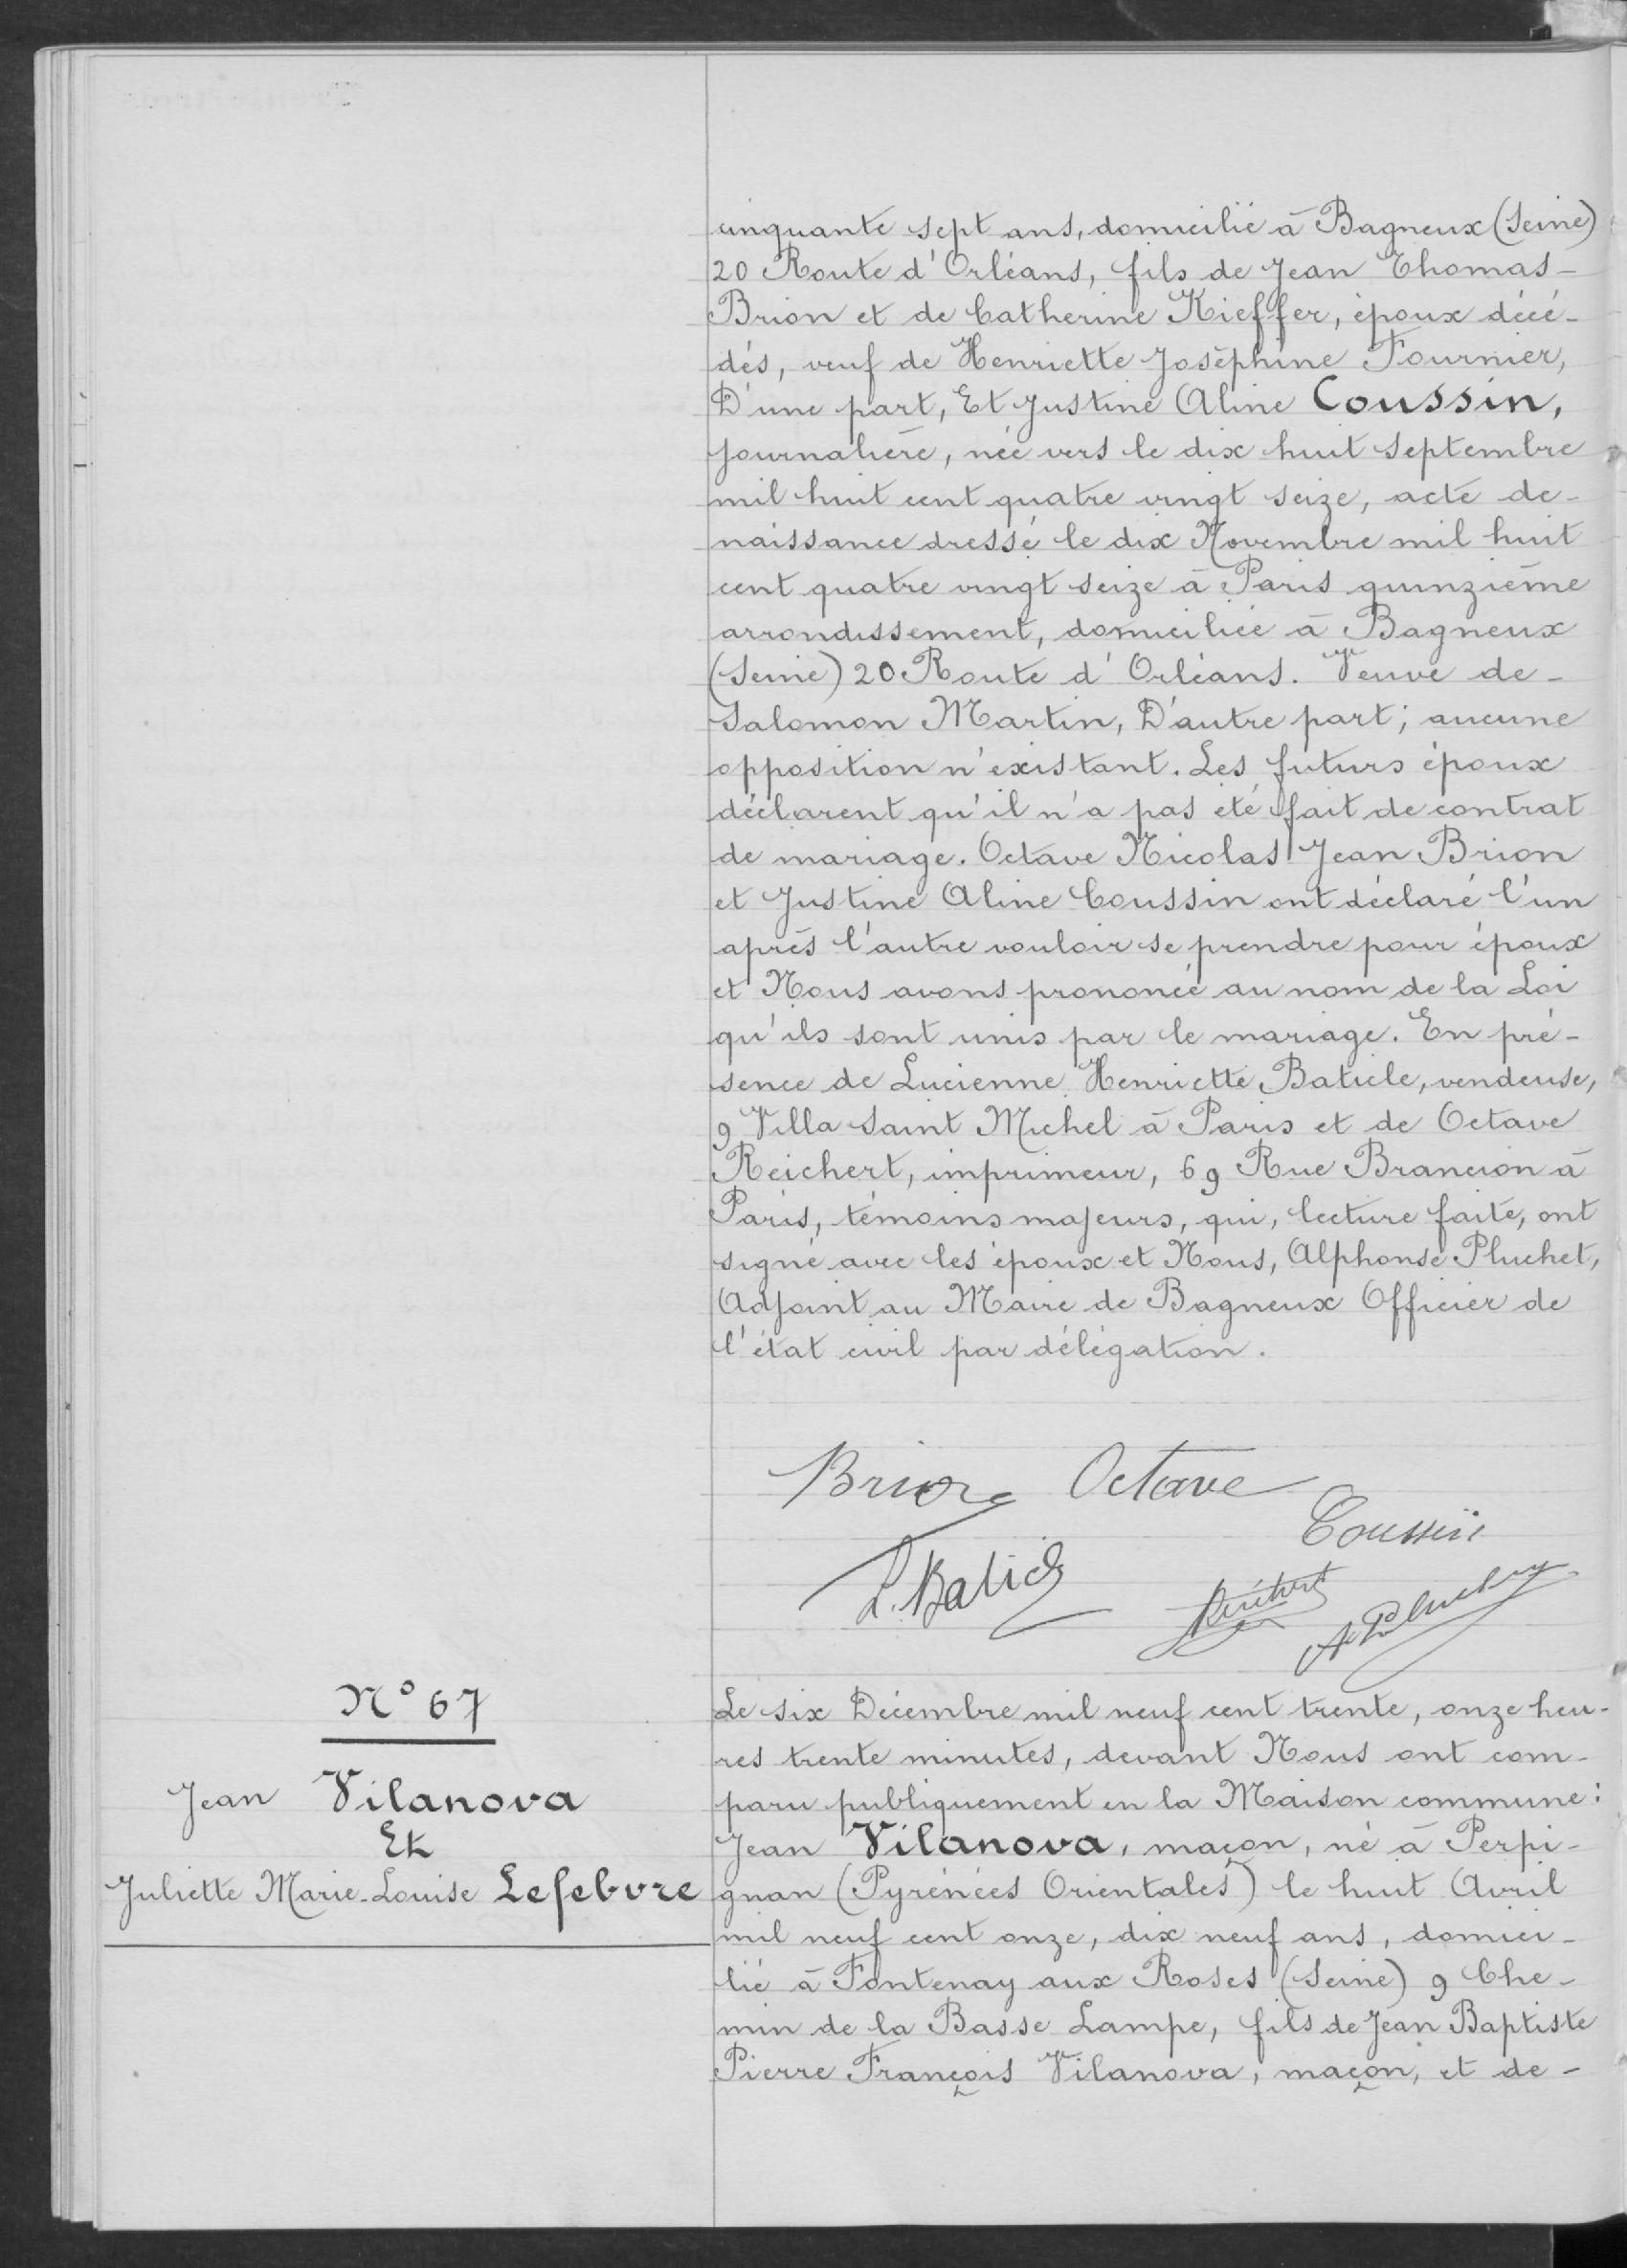cinquante sept ans, domicilié à Bagneux (Seine)
20 Route d'Orléans, fils de Jean Thomas -
Brion et de Catherine Kieffer, époux déc-
dés, veuf de Henriette Joséphine Fournier,
D'une part, Et Justine Aline Coussin,
journalière, née vers le dix-huit septembre
mil huit cent quatre vingt seize, acté de
naissance dressé le dix Novembre mil huit
cent quatre vingt seize à Paris quinzième
arrondissement, domiciliée à Bagneux
(Seine) 20 Route d'Orléans. Veuve de -
Salomon Martin, D'autre part; aucune
opposition n'existant. Les futurs époux
déclarent qu'il n'a pas été fait de contrat
de mariage. Octave Nicolas Jean Brion
et Justine Aline Coussin ont déclaré l'un
après l'autre vouloir se prendre pour époux
et Nous avons prononcé au nom de la Loi
qu'ils sont unis par le mariage. En pré-
sence de Lucienne Henriette Batrile, vendeuse,
9 Villa Saint Michel à Paris et de Octave
Reichert, imprimeur, 69 Rue Brancion à
Paris, témoins majeurs, qui, lecture faite, ont
signé avec les époux et Nous, Alphonse Pluchet
Adjoint au Maire de Bagneux Officier de
l'état civil par délégation
20 Route d'Orléans, fils de Jean Thomas -
Brion et de Catherine Kieffer, époux déc-
dés, veuf de Henriette Joséphine Fournier,
D'une part, Et Justine Aline Coussin,
journalière, née vers le dix-huit septembre
mil huit cent quatre vingt seize, acté de
naissance dressé le dix Novembre mil huit
cent quatre vingt seize à Paris quinzième
arrondissement, domiciliée à Bagneux
(Seine) 20 Route d'Orléans. Veuve de -
Salomon Martin, D'autre part; aucune
opposition n'existant. Les futurs époux
déclarent qu'il n'a pas été fait de contrat
de mariage. Octave Nicolas Jean Brion
et Justine Aline Coussin ont déclaré l'un
après l'autre vouloir se prendre pour époux
et Nous avons prononcé au nom de la Loi
qu'ils sont unis par le mariage. En pré-
sence de Lucienne Henriette Batrile, vendeuse,
9 Villa Saint Michel à Paris et de Octave
Reichert, imprimeur, 69 Rue Brancion à
Paris, témoins majeurs, qui, lecture faite, ont
signé avec les époux et Nous, Alphonse Pluchet
Adjoint au Maire de Bagneux Officier de
l'état civil par délégation
N°67
Jean Vilanova
Et
Juliette Marie Louise Lefebvre
Le six Décembre mil neuf cent trente, onze heu-
res trente minutes, devant Nous ont com-
paru publiquement en la Maison commune:
Jean Vilanova, maçon, né à Perpi-
gnan (Pyrénes Orientales) le huit Avril
mil neuf cent onze, dix neuf ans, domici-
lié à Fontenay aux Roses (Seine) 9 Che-
min de la Basse Lampe, fils de Jean Baptiste
Pierre François Vilanova, maçon, et de-
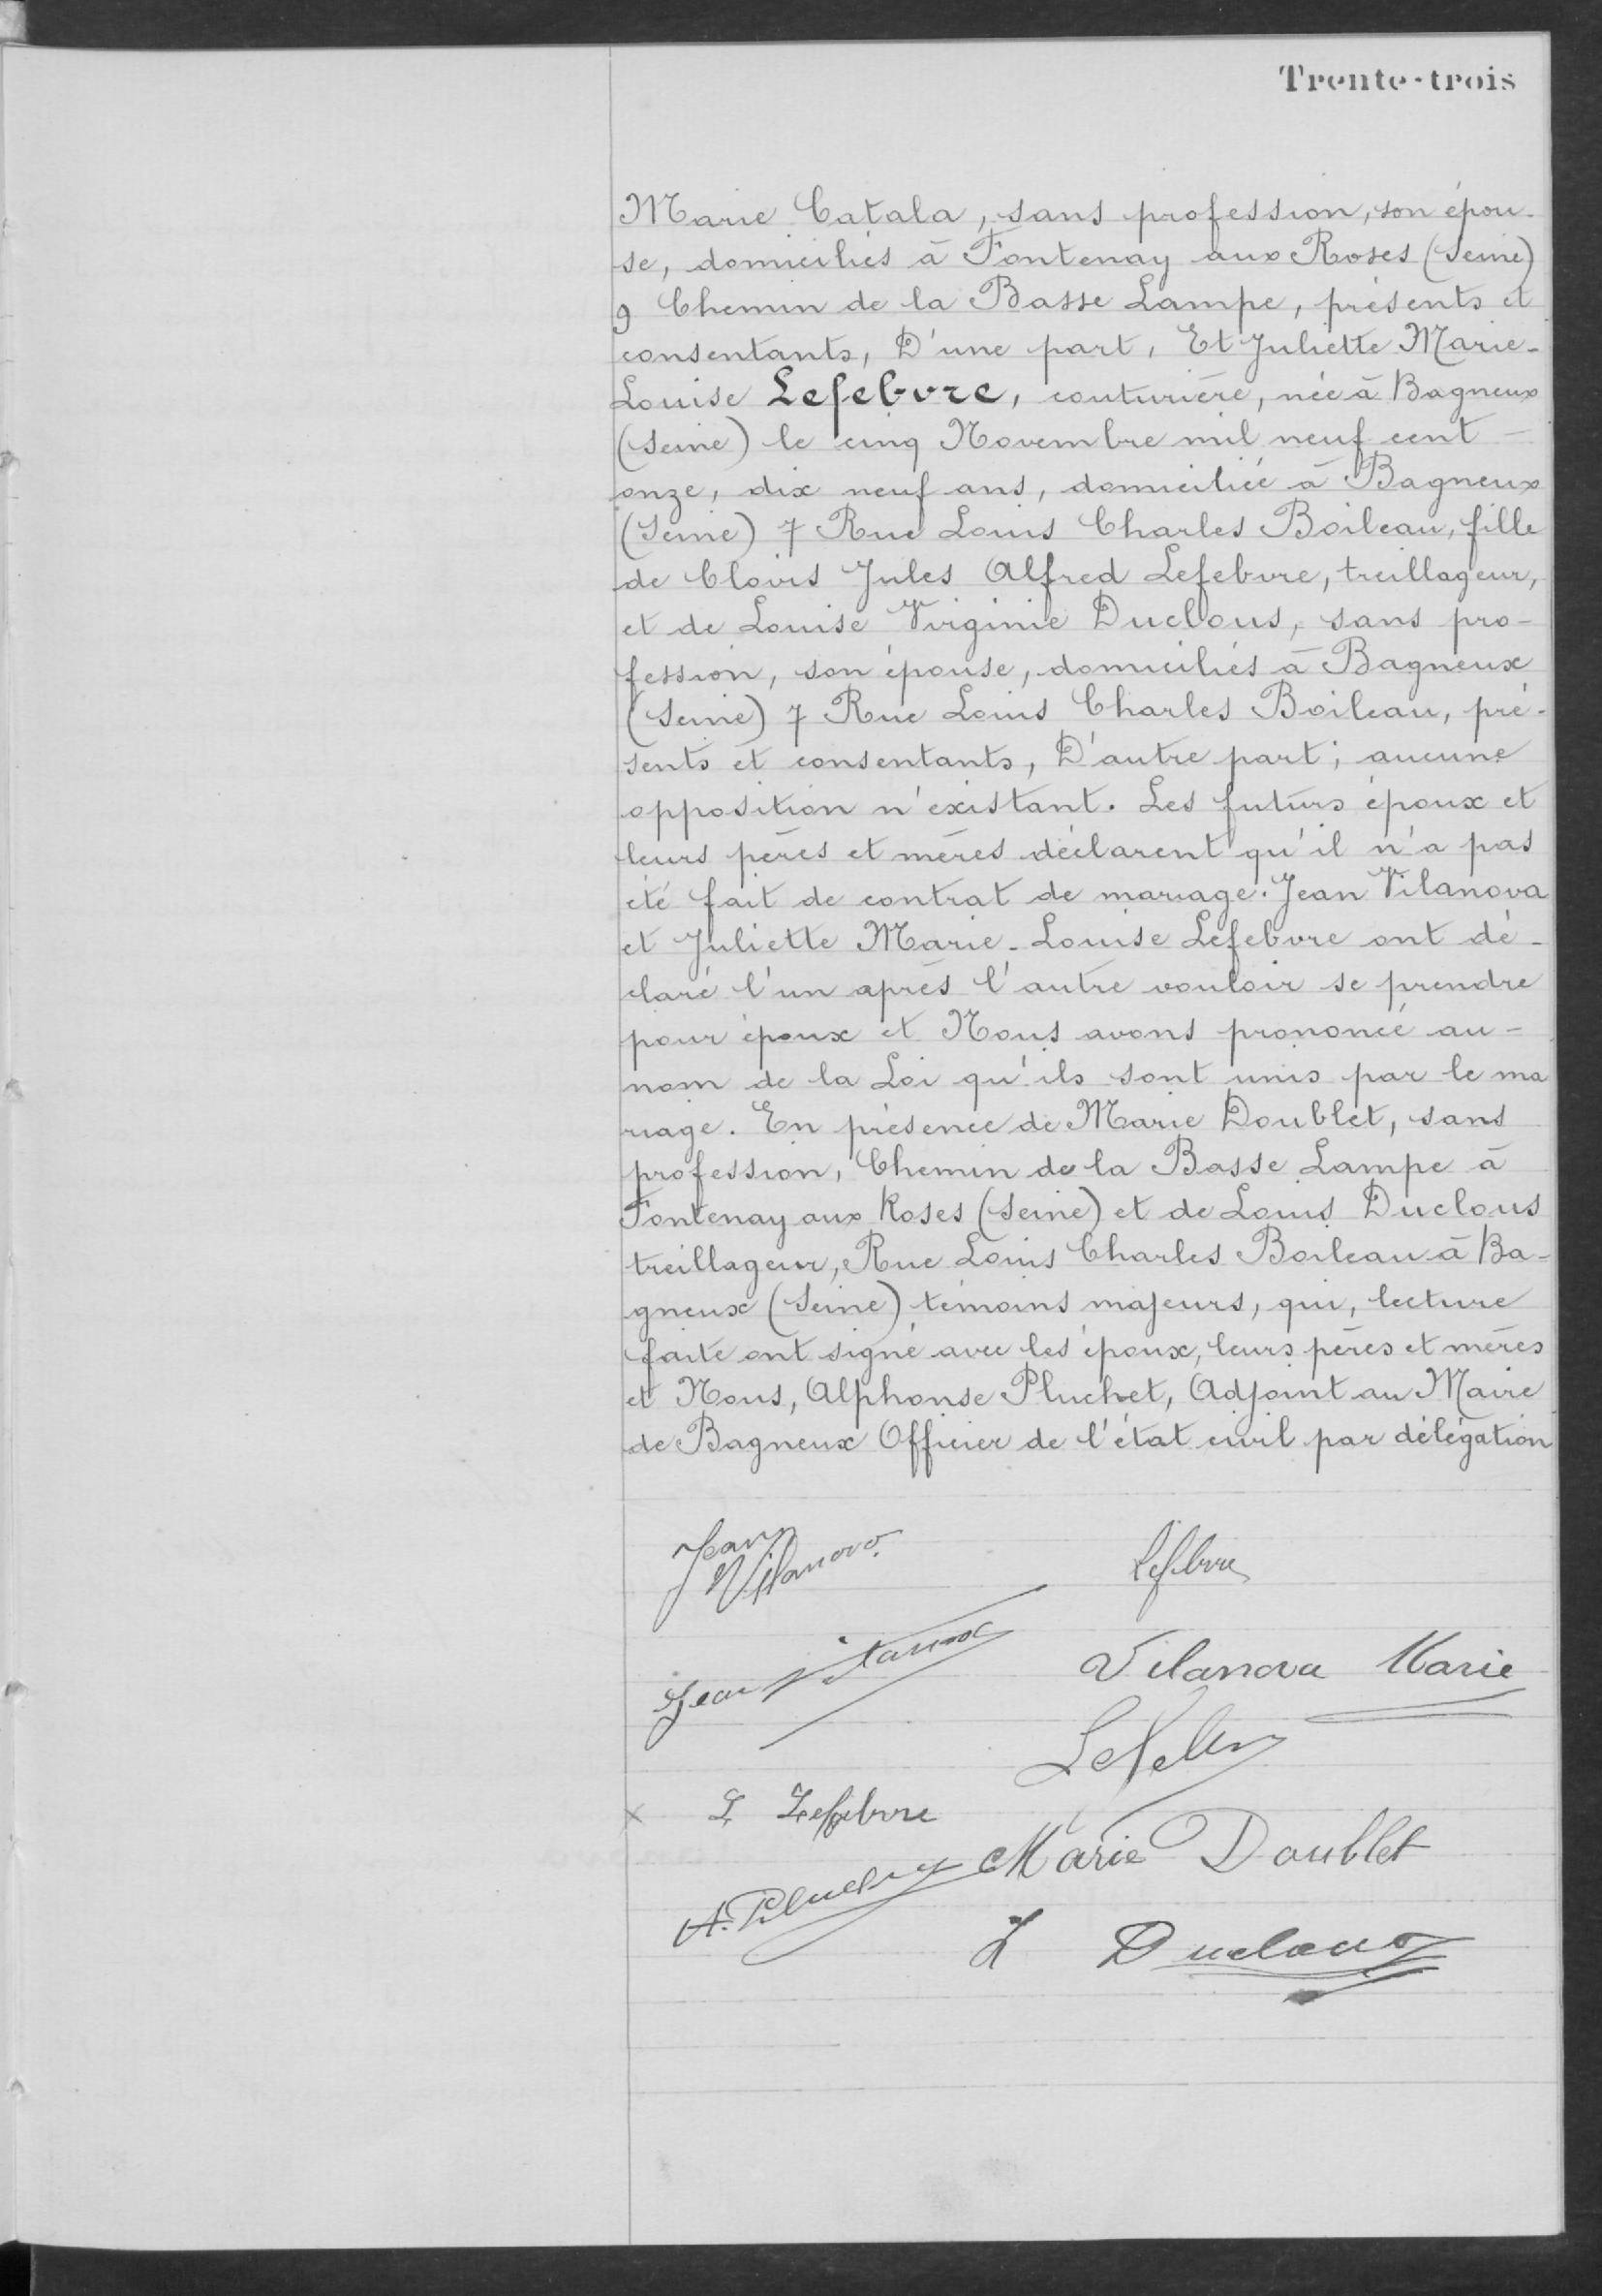Madame Catala, sans profession, son épou-
se, domiciliés à Fontenay aux Roses (Seine)
9 Chemin de la Basse Lampe, présents et
consentants, d'une part, Et Juliette Marie-
Louise Lefebvre, couturière, née à Bagneux
(Seine) le cinq Novembre mil neuf cent-
onze, dix neuf ans domiciliée à Bagneux
(Seine) 7 Rue Louis Charles Boileau, fille
de Clovis Jules Alfred Lefebvre, treillageur,
et de Louise Virginie Duellons, sans pro-
fession, son épouse, domiciliés à Bagneux
(Seine) 7 Rue Louis Charles Boileau, pré-
sents et consentants, D'autre part: aucune
opposition n'existant. Les futurs époux et
leurs pères et mères déclarent qu'il n'a pas
été fait de contrat de mariage. Jean Vilanova
et Juliette Marie-Louise Lefebvre ont dé-
claré l'un après l'autre vouloir se prendre
pour époux et Nous avons prononcé au-
nom de la Loi qu'ils sont unis par le ma
riage. En présence de Marie Doublet, sans
profession, Chemin de la Basse Lampe à
Fontenay aux Roses (Seine) et de Louis Duclons
treillageur, Rue Louis Charles Boileau à Ba-
gneux (Seine), témoins majeurs, qui, lecture
faite ont signé avec les époux, leurs pères et mères
et Nous, Alphonse Pluelvet, Adjoint au Maire
de Bagneux Officier de l'état civil par délégation